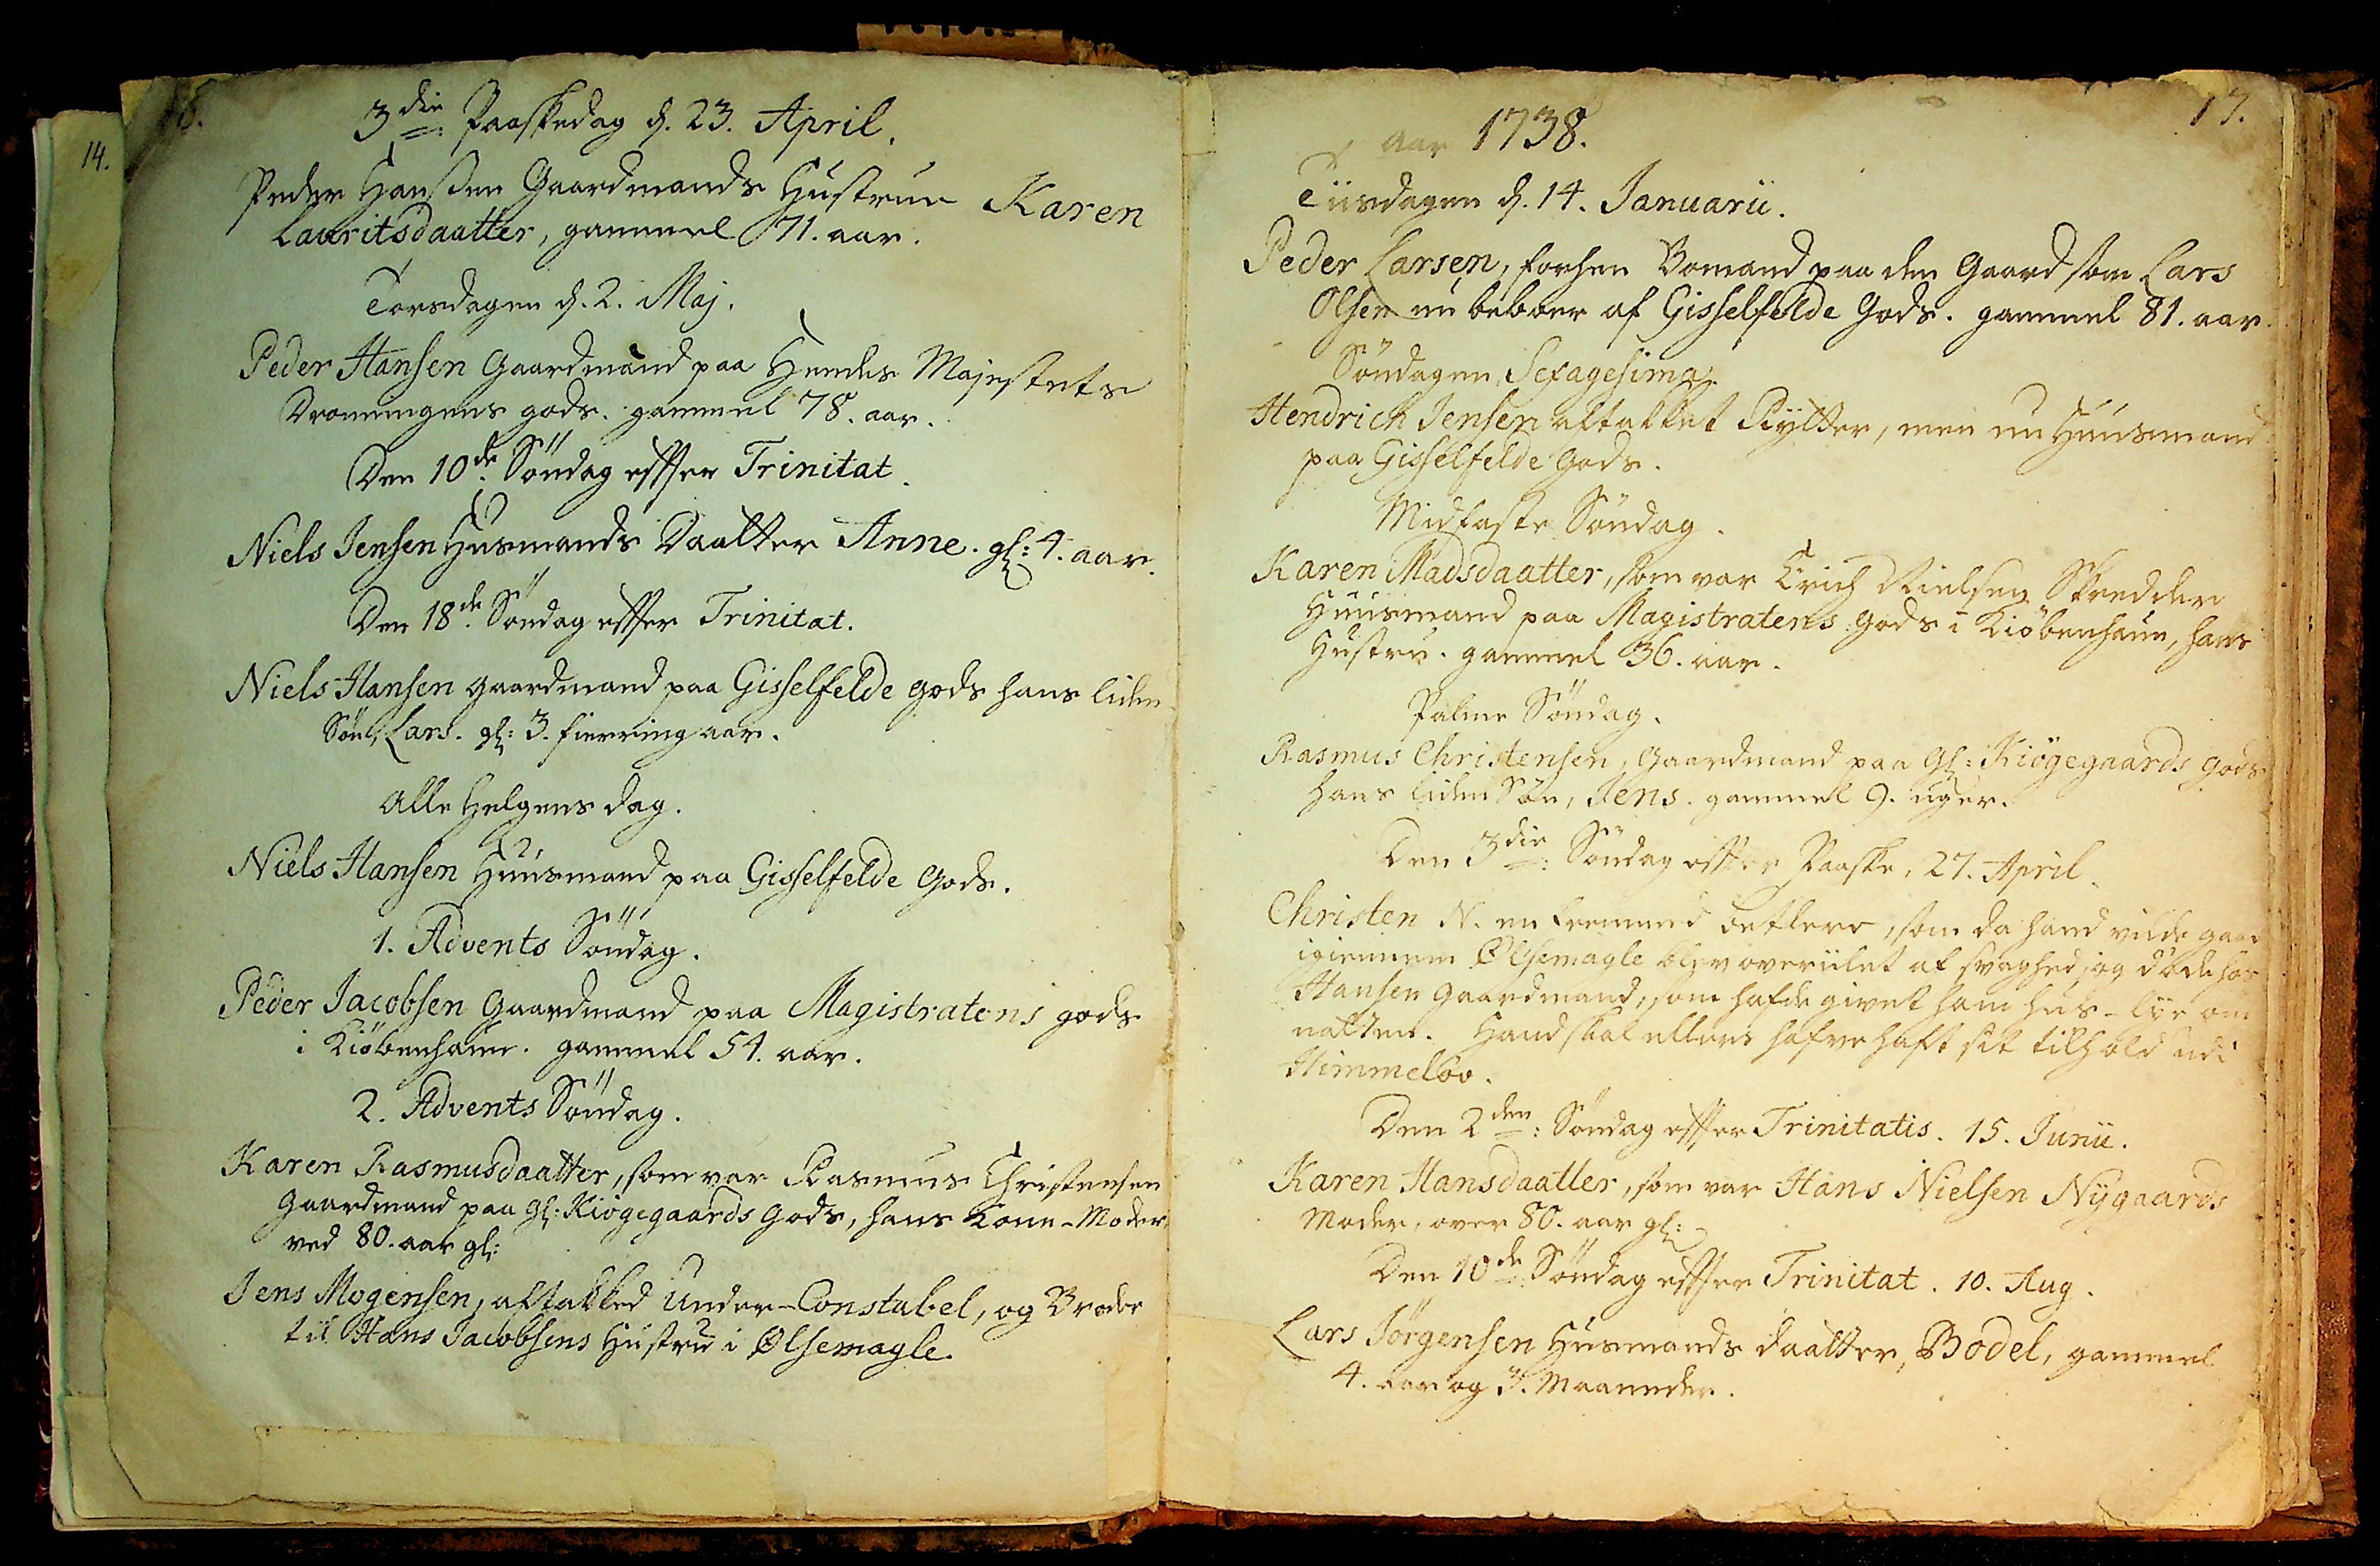16.
3die: Paaskedag d. 23. April.
Peder Hansen Gaardmands Hustrue Karen
 Lauritsdaatter, gammel 71. aar.
Torsdagen d. 2. Maj.
Peder Hansen Gaardmand paa Hendes Majestets
Dronningens gods. gammel 78. aar.
Den 10de Søndag efter Trinitat.
Niels Jensen Husmands Daatter Anne. gl: 4. aar.
Den 18de Søndag efter Trinitat.
Niels Hansen Gaardmand paa Gisselfelde Gods hans liden
Søn, Lars. gl: 3. fierring aar.
Alle Helgens dag.
Niels Hansen Huusmand paa Gisselfelde Gods.
1. Advents Søndag.
Peder Jacobsen Gaardmand paa Magistratens gods
i Kiøbenhavn. gammel 54. aar.
2. Advents Søndag.
Karen Rasmusdaatter, som var Rasmus Christensen
Gaardmand paa Gl: Kiøgegaards Gods, hans Kone-Moder
ved 80. aar gl:
Jens Mogensen, aftakked Under-Constabel, og Broder
til Hans Jacobsens Hustru i Ølsemagle.
17.
Aar 1738.
Tirsdagen d. 14. Januarii.
Peder Larsen, forhen Bomand paa den Gaard som Lars
Olsen nu beboer af Gisselfelde Gods. gammel 81. aar.
Søndagen, Sexagesima.
Hendrich Jensen aftakket Rytter, men nu Huusmand
paa Gisselfelde Gods.
Midfaste Søndag.
Karen Madsdaatter, som var Erich Nielsen Skredder
Huusmand paa Magistratens Gods i Kiøbenhavn, hans
Hustru. gammel 36. aar.
Palme Søndag
Rasmus Christensen, Gaardmand paa Gl: Kiøgegaards Gods
hans liden Søn, Jens. gammel 9. uger.
Den 3die: Søndag efter Paaske, 27. April.
Christen N. en fremmed Betlere, som da hand vilde gaa
igiennem Ølsemagle blev overiilet af svaghed; og døde hos
Hansen Gaardmand, som havde givet ham hus-lye om
natten. Hand skal ellers hafve haft sit tilhold udi
Himmeløv.
Den 2den: Søndag efter Trinitatis. 15. Junii.
Karen Hansdaatter, som var Hans Nielsen Nygaards
Moder, over 80. aar gl:
Den 10de: Søndag efter Trinitat. 10. Aug.
Lars Jørgensen Husmands daatter, Bodel, gammel
4. aar og 3. Maaneder. 
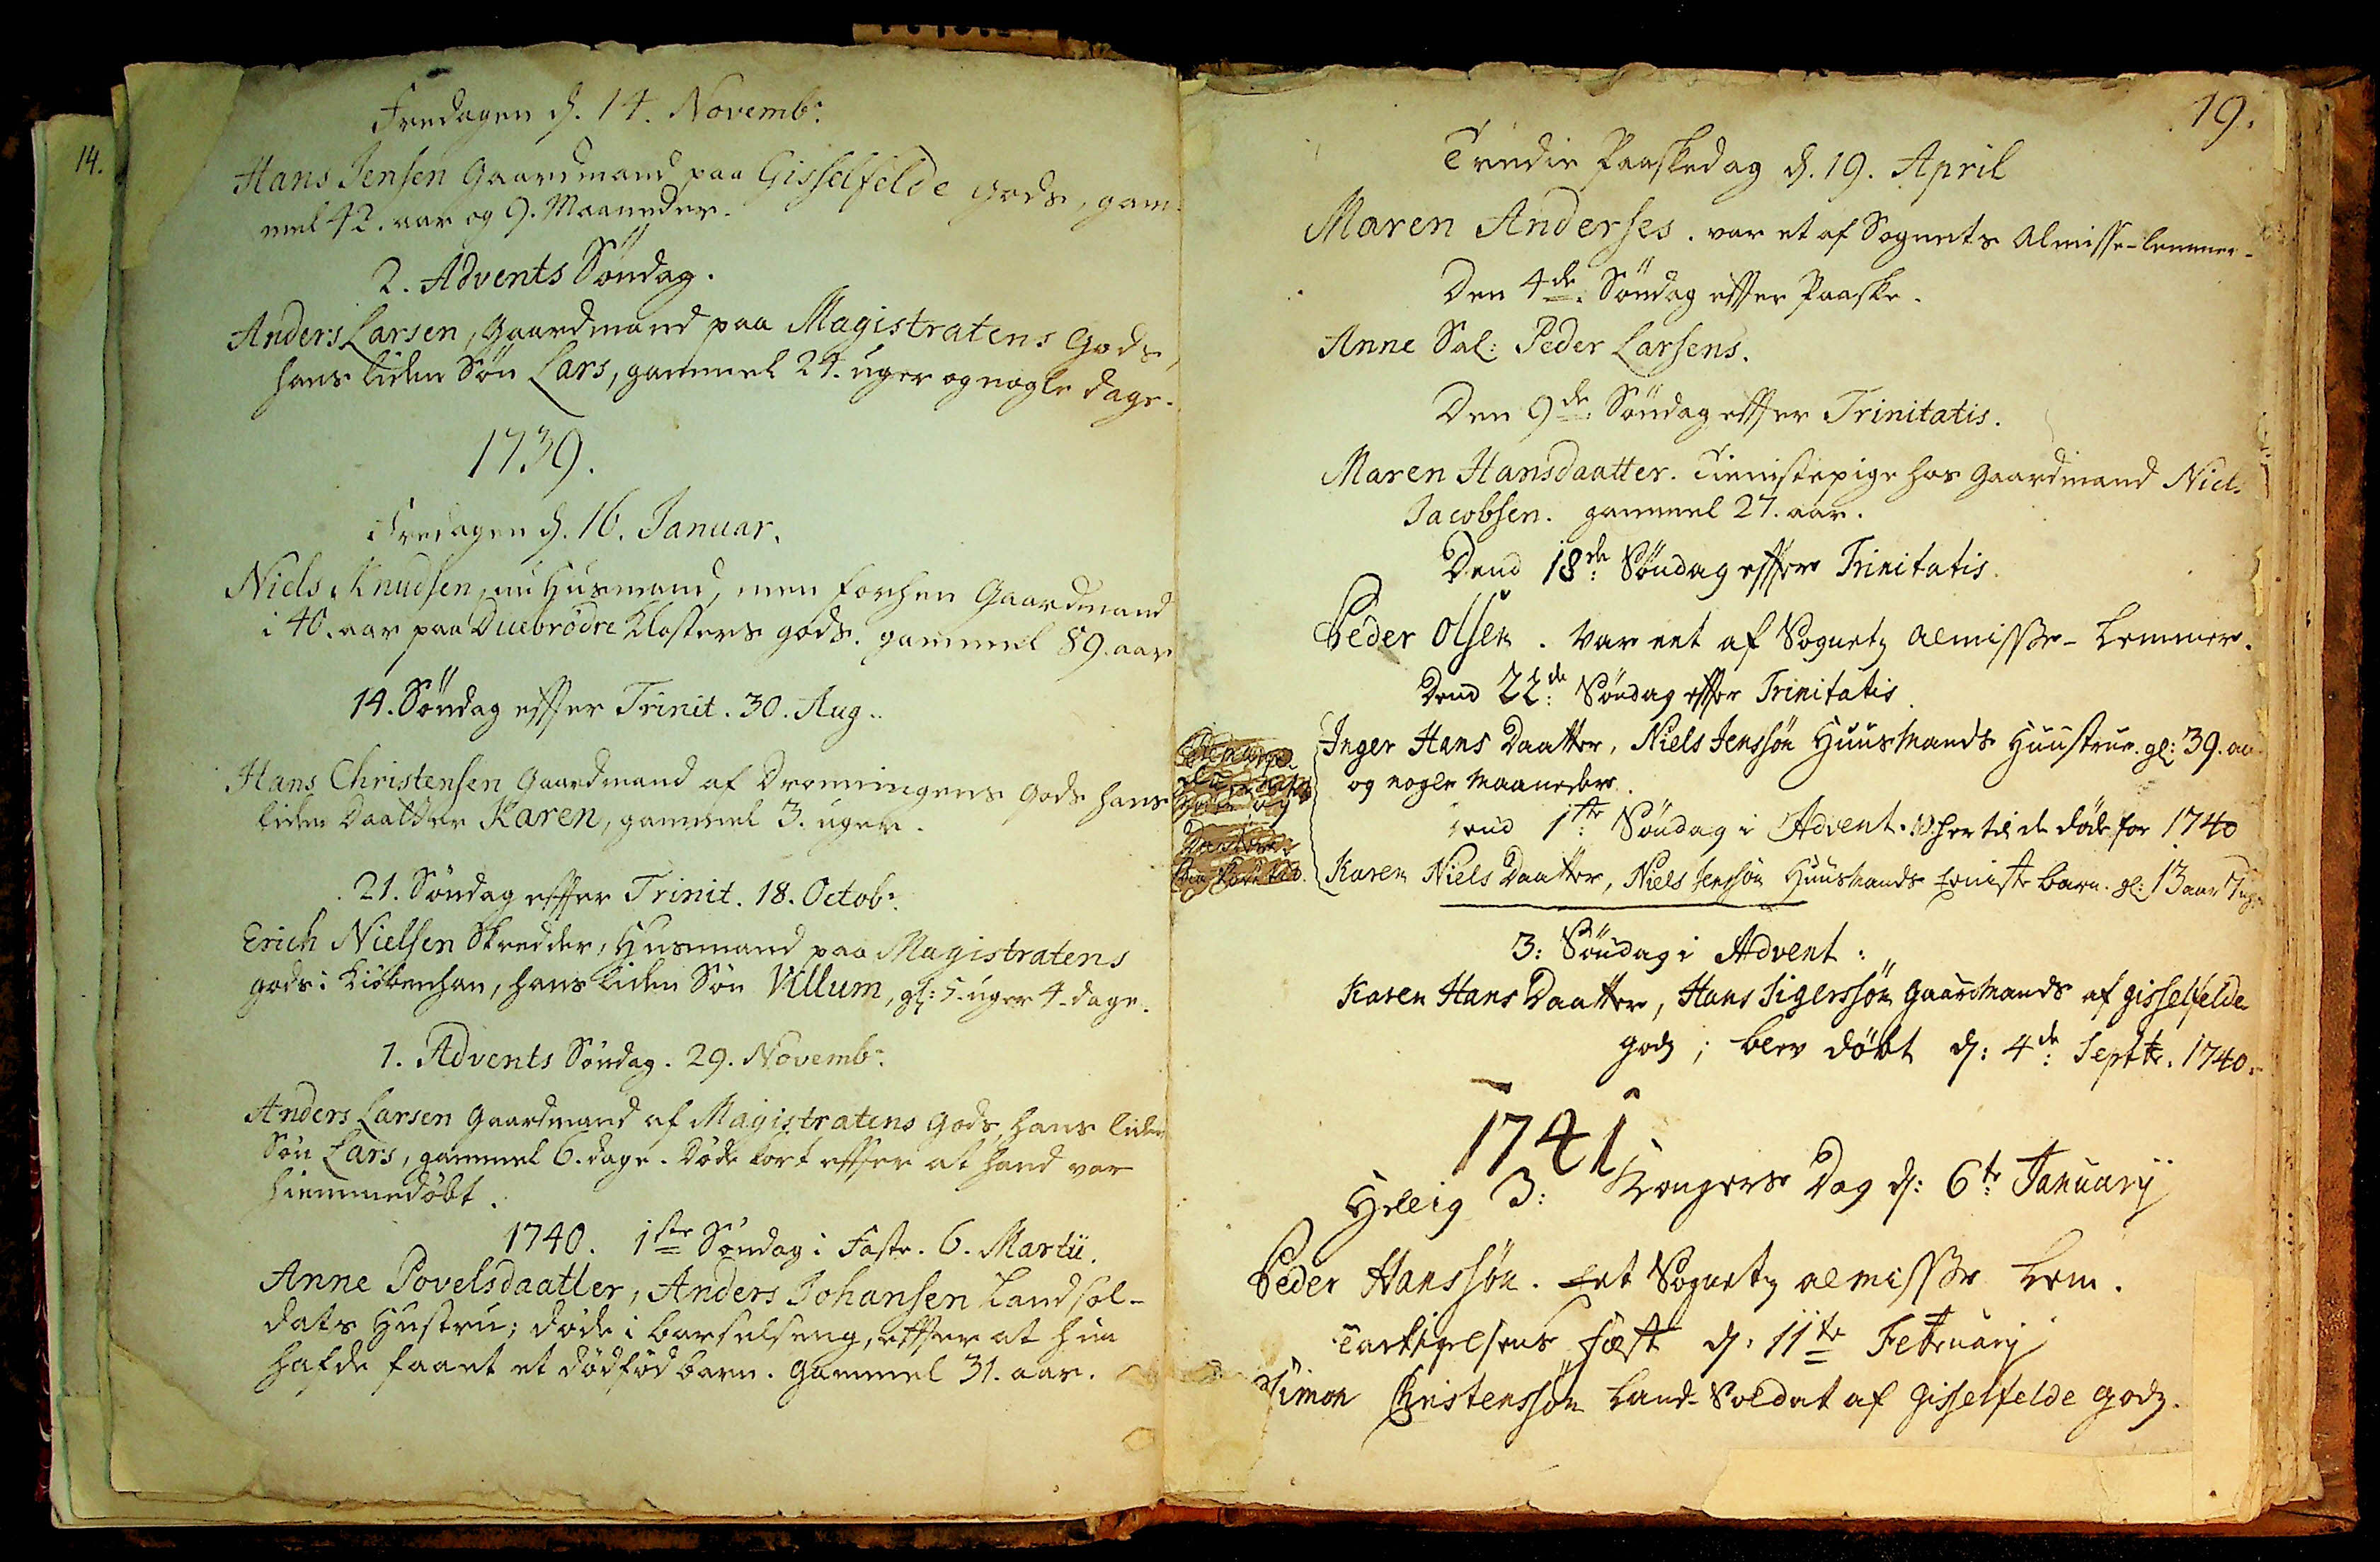Fredagen d. 14. Novembr:
Hans Jensen Gaardmand paa Gisselfelde Gods, gam
mel 42. aar og 9. Maaneder.
2. Advents Søndag.
Anders Larsen, Gaardmand paa Magistratens Gods,
hans liden Søn Lars, gammel 24. uger og nogle dage.
1739.
Fredagen d. 16. Januar.
Niels Knudsen, en Husmand, men forhen Gaardmand
i 40. aar paa Duebrødre Klosters Gods. gammel 89. aar.
14. Søndag efter Trinit. 30. Aug.
Hans Christensen Gaardmand af Dronningens Gods hans 
liden Daatter Karen, gammel 3. uger. 
21. Søndag efter Trinit. 18. Octobr.
Erich Nielsen Skredder, Husmand paa Magistratens
Gods i Kiøbenhan, hans liden Søn Villum, gl: 5.uger 4.dage.
1. Advents Søndag. 29. Novembr:
Anders Larsen Gaardmand af Magistratens Gods, hans liden
Søn Lars, gammel 6.dage. Døde kort efter at hand var
hiemmedøbt.
1740.1ste Søndag i Faste. 6. Martii.
Anne Povelsdaatter, Anders Johansen Landsol¬
dats Hustru; døde i barselseng, efter at hun
hafde faaet et dødfød barn. Gammel 31. aar.
19.
Tredie Paaskedag d. 19. April
Maren Anderses. var et af Sognets Almisselemmer.
Den 4de. Søndag efter Paaske.
Anne Sal: Peder Larsens.
Den 9de: Søndag efter Trinitatis.
Maren Hansdaatter. Tienestepige hos Gaardmand Niels
Jacobsen. gammel 27. aar.
Dend 18de: Søndag efter Trinitatis.
Peder Olsen. var et af Sognets Almisse-Lemmer
Dend 22de: Søndag efter Trinitatis.
Inger Hans Daatter, Niels Jenssøn Huusmands Huustrue. gl: 39.aar
og nogle Maaneder.
Dend 1ste: Søndag i Advent. NB. her til de døde for 1740
Karen Niels Daatter, Niels Jenssøn Huusmands Eeniste barn. gl:13aar 7 Uger
3: Søndag i Advent:
Karen Hans Daatter, Hans Sigerssøn Gaardmands af Gisselfelde
Gods; blev døbt d: 4de: Septbr: 1740
1741
Hellig 3: Kongers Dag d: 6te: Januarij
Peder Hanssøn. Eet Sognets almisse Lem.
Taeksigelsens Fæst d: 11te Februarij
Simon Christenssøn Land-Soldat af Gisselfelde Godz.